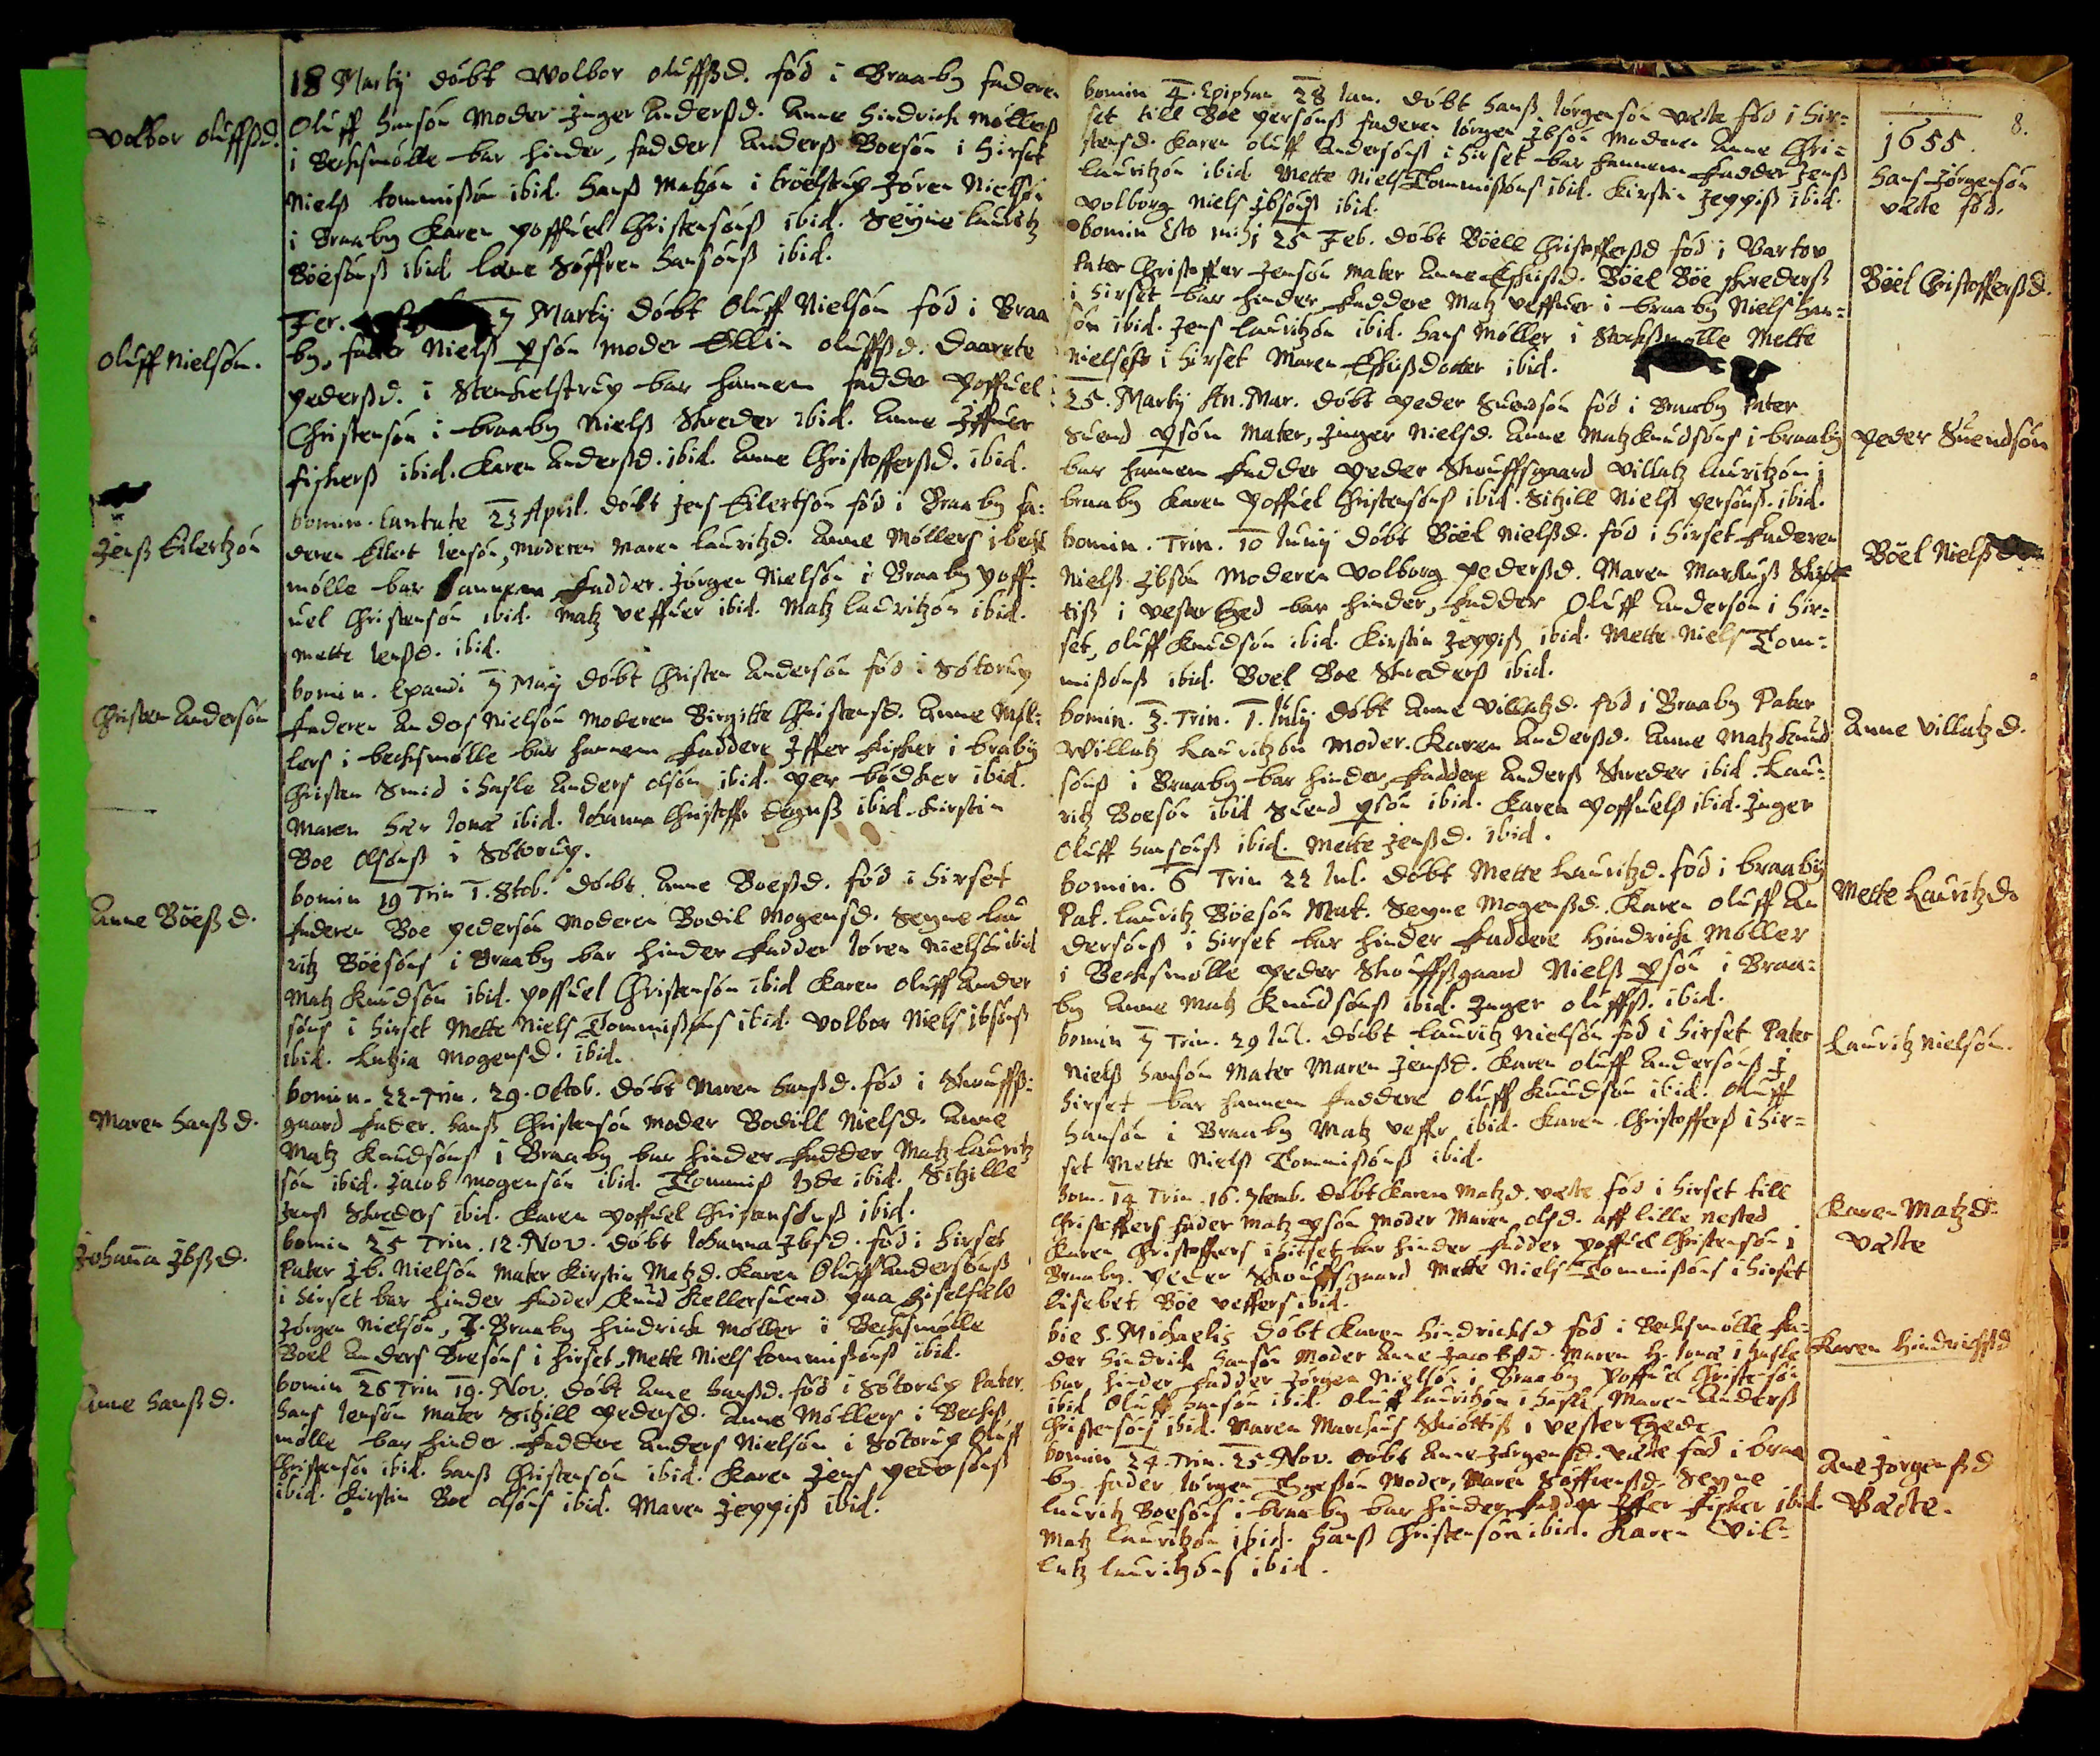Valbor Oluffsd.
18. Marty døbt Volbor Oluffsd. fød i Braaby Fader
Oluff Hansøn Moder Jnger Andersd. Anne Hindrick Møllers
i Becksmølle bar hinder, Fadder Anders Boesøn i Hirset.
Niels Tommisøn ibid. Hans Matzøn i Troelstrup. Jøren Nielsøn
i Braaby. Karen Poffuel Christensøns ibid. Seigne Lauritz
Boesøns ibid. Læne Søffren Hansøns ibid.
Oluff Nielsøn 
Fer. Post Pascha## 7. Marty døbt Oluff Nielsøn. fød i Braa
by. Fader Niels Psøn. Moder Ellin Oluffsd. Daarete
Pedersd. i Stenkelstrup bar hannem Fadder Poffuel
Christensøn i Braaby. Niels skreder ibid. Anne Jffuer
Fiskers ibid. Karen Andersd. ibid. Anne Christoffersd. ibid.
Jens Eilertzøn 
Domin Cantate 23 April døbt Jens Eilertsøn fød i Braaby Fa¬
deren Eilert Jensøn, Moderen Maren Lauritzd. Anne Møllers i Becks
mølle bar hannem, Fadder: Jørgen Nielsøn i Braaby. Poff¬
uel Christensøn ibid. Matz Veffuer ibid. Matz Lauritzøn ibid.
Mette Jensd. ibid.
Christen Andersøn 
Domin Exaudi 7. May døbt Christen Andersøn fød i Sø Torup.
Faderen Anders Nielsøn Moderen Birgitte Christensd. Anne Møl¬
lers i Becksmølle bar hannem. Faddere: Jffer Fisker i Brabÿ
Christen Smid i Hasle Anders Olsøn ibid. Per Bødker ibid.
Maren Hær Jonæ ibid. Johanna Christoffer Degns ibid. Kirstin
Boe Olsøns i Søtorup.
Anne Boesd. 
Domin 19 Trin 1. 8tob. døbt Anne Boesd. fød i Hirset.
Faderen Boe Pedersøn Moderen Bodil Mogensd. Seigne Lau
ritz Boesøns i Braaby bar hinder. Fadder: Jøren Nielsøn ibid
Matz Knudsøn ibid. Poffuel Christensøn ibid. Karen Oluff Ander
søns i Hirset Mette Niels Tommisøns ibid. Valbor Niels Ibsøns 
ibid. Lutzia Mogensd. ibid.
Maren Hansd. 
Domin 22. Trin. 29. Octob. døbt Maren Hansd. fød i Skouffs¬
gaard Fader. Hans Christensøn Moder Bodill Nielsd. Anne
Matz Knudsøns i Braaby bar hinder Fadder Matz Lauritz
søn ibid. Jacob Mogensøn ibid. Tommis Jyde ibid. Sitzille
Jens Skreders ibid. Karen Poffuel Christensøns ibid.
Johanna Jbsd. 
Domin 25 Trin. 12. Nov. døbt Johanna Jbsd. fød i Hirset
Pater Jb Nielsøn. Mater Kirstin Matsd. Karen Oluff Andersøns
i Hirset bar hinder. Fadder: Knud Kellersuend paa Giselfæls
Jørgen Nielsøn, J Braaby. Hindrick Møller i Becksmølle
Boel Anders Boesøns i Hirset, Mette Niels Tommisøns ibid.
Anne Hansd.
Domin 26 Trin 19. Nov. døbt Anne Hansd. i Søtorup. Pater
Hans Jensøn Mater Sitzill Pedersd. Anne Møllers i Becks¬
mølle bar hinder Faddere Anders Nielsøn i Søtorup. Oluff
Christensøn ibid. Hans Christensøn ibid. Karen Jens Pedersøns
ibid. Kirstin Boe Olsøns ibid. Maren Jeppis ibid.
Domin 4. Epiphan. 28. Jan. døbt Hans Jørgensøn uæcte fød i Hir¬
set till Boe Persøns Faderen Jørgen Jbsøn. Moderen Anne Chri¬
stensd. Karen Oluff Andersøns i Hirset bar hannem Fadder Jens
Lauritzøn ibid. Mette Niels Tommissøns ibid. Kirstin Jeppis ibid.
Valborg Niels Jbsøns ibid.
8.
1655
Hans Jørgensøn 
Uæcte fød.
Domin Esto Mihi 25 Feb. døbt Boell Christoffersd. fød i Vartov
Pater Christoffer Jensøn Mater Anne Eskisd. Boel Boe Skreders
i Hirset bar hinder, Faddere Matz Veffuer i Braaby Niels Han¬
søn ibid. Jens Lauritzøn ibid. Hans Møller i Stocksmølle Mette
Nielsis i Hirset Maren Eskisdotter ibid.
Boel Christoffersd.
25 Marty An. Mar. døbt Peder Suendsøn fød i Braaby Pater
Suend Psøn. Mater, Jnger Nielsd. Anne Matz Knudsøns i Braaby
bar hannem Fadder Peder Skouffsgaard Villatz Lauritzøn i
Braaby Karen Poffuel Christensøns ibid. Sitzill Niels Persøns ibid.
Peder Suendsøn
Domin. Trin. 10 Juny døbt Boel Nielsd. fød i Hirset. Faderen
Niels Jbsøn. Moderen Volborg Pedersd. Maren Markus Skiøt¬
tis i Vester Eged bar hinder, Fadder Oluff Andersøn i Hir¬
set, Oluff Knudsøn ibid. Kirstin Jeppis ibid. Mette Niels Tom¬
misøns ibid. Boel Boe Skreders ibid.
Boel Nielsdot.
Domin. 3. Trin. 1. July døbt Anne Villatzd. fød i Braaby Pater
Villatz Lauriztøn Moder Karen Andersd. Anne Matz Knud
søns i Braaby bar hinder, Faddere Anders Skreder ibid. Lau¬
ritz Boesøn ibid. Suend Psøn ibid. Karen Poffuels ibid. Jnger
Oluff Hansøns ibid. Mette Jensd. ibid.
Anne Villatzd.
Domin. 6 Trin. 22 Jul. døbt Mette Lauritzd. fød i Braaby
Pat. Lauritz Boesøn Mat. Segne Mogensd. Karen Oluff An
dersøns i Hirset bar hinder Faddere: Hendrick Møller
i Becksmølle Peder Skouffsgaard Niels Psøn i Braa¬
by. Anne Matz Knudsøns ibid. Jnger Oluffs ibid.
Mette Lauritzd.
Domin 7 Trin 29. Jul. døbt Lauritz Nielsøn fød i Hirset, Pater
Niels Hansøn Mater Maren Jensd. Karen Oluff Andersøns J
Hirset bar hannem. Faddere Oluff Knudsøn ibid. Oluff
Hansøn i Braaby, Matz Veffer ibid. Karen Christoffers i Hir¬
set Mette Niels Tommisøns ibid.
Lauritz Nielsøn 
Dom. 14 Trin. 16. 7temb. døbt Karen Matzd. uæcte fød i Hirset till
Christoffers Fader Mats Psøn Moder Maren Olsd. aff Lille Nested
Karen Christoffers i Hirset bar hinder Fadder Poffuel Christensøn i
Braaby. Peder Skouffsgaard Mette Niels Tommisøns i Hirset
Lisebet Boe Veffers ibid.
Karen Matzd.
Uæcte 
Die S. Michaelis døbt Karen Hindricksd. fød i Becksmølle Fa¬
der Hindrick Hansøn Moder Anne Jacobsd. Maren H. Jonæ i Hasle 
bar hinder. Fadder Jørgen Nielsøn i Braaby Poffuel Christensøn
ibid Oluff Hansøn ibid. Oluff Lauritzøn i Hasle Maren Anders
Christensøns ibid. Maren Marcheus Skiøttis i Vester Egede
Karen Hindricksd.
Domin 24. Trin 25. Nov. døbt Anne Jørgensd. Uæcte fød i Braa
by. Fader Jørgen Tyesøn## Moder, Maren Søffrensd. Segne
Lauritz Boesøns i Braaby bar hinder Fadder Iffer Fisker ibid.
Matz Lauritzøn ibid. Hans Christensøn ibid. Karen Vil¬
latz Lauritzdot. ibid.
Ane Jørgensd 
Uæcte.